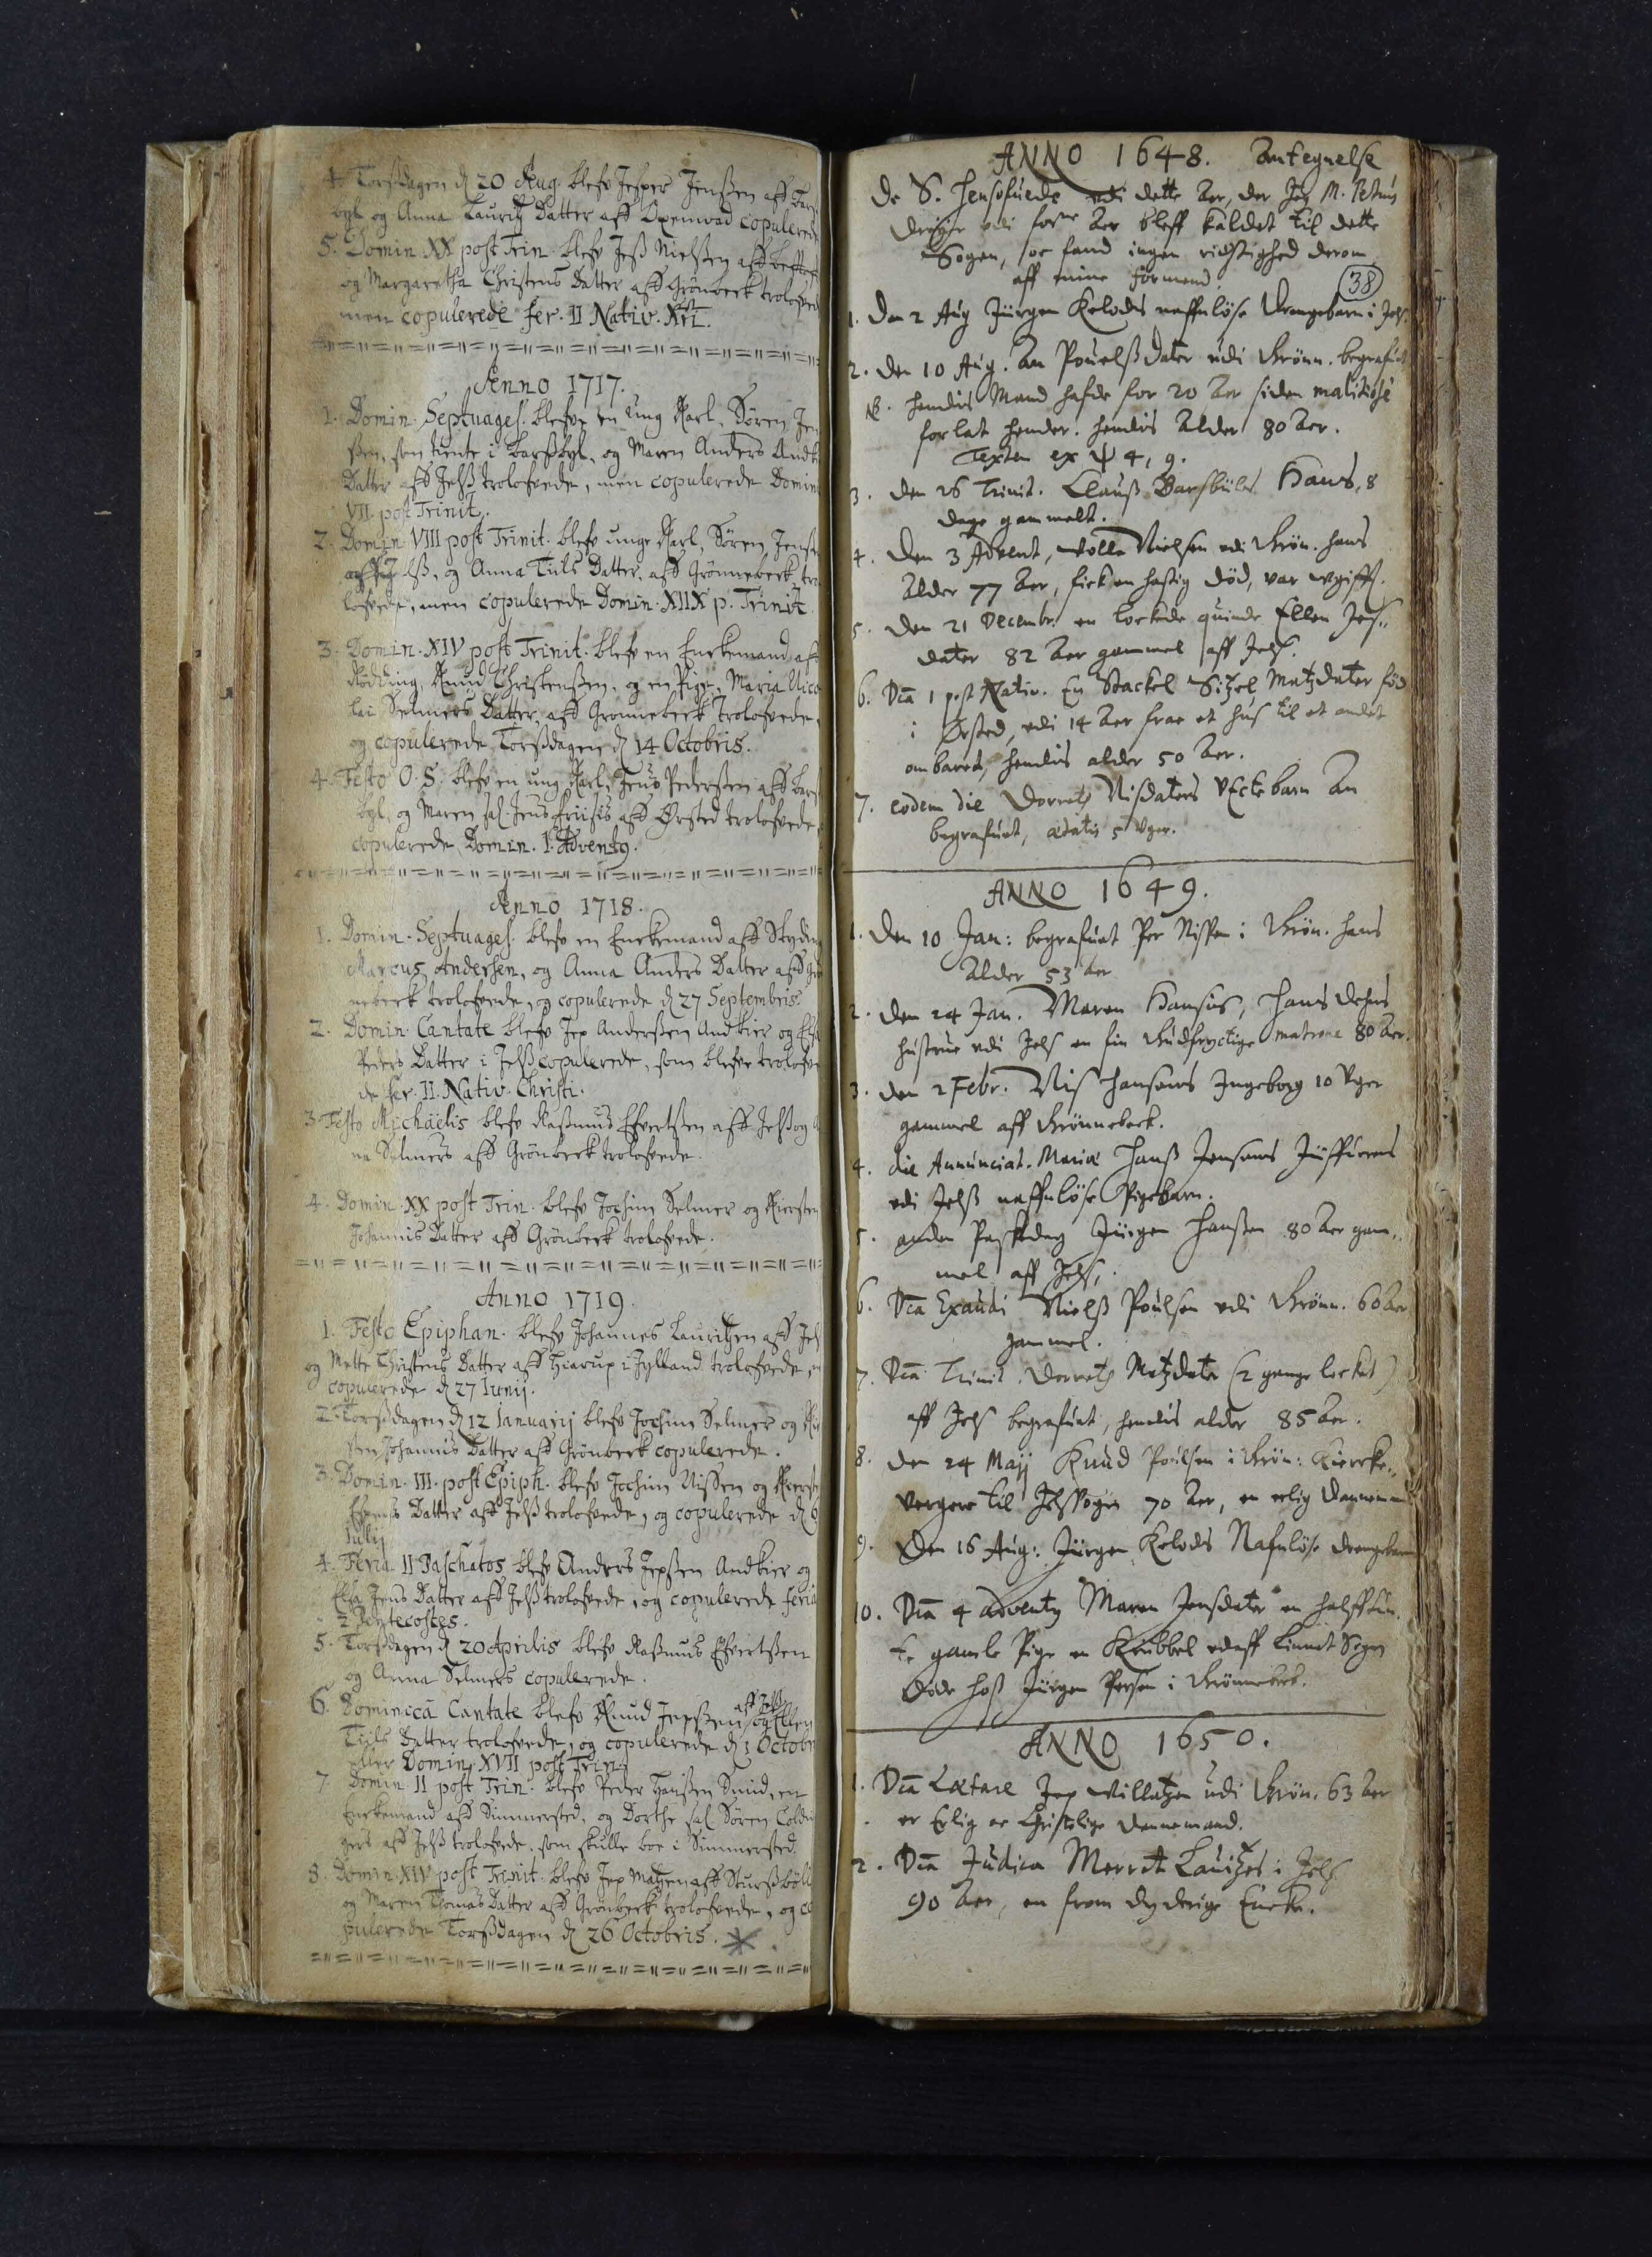4. Torssdagen d 20 Aug. blefv Jesper Jenssen aff Bars
byl og Anna Lauritz Datter aff Oxemvad copulerede
5. Domin. XX post Trin blefv Jess Nielssen aff Befftoft
og Margaretha Christens Datter aff Grønbeck trolofede
men copulerede fer. II Nativ. Xti.
Anno 1717.
1. Domin. Septuages. Blefve en ung Karl, Søren Jen
ssen, som tiente i Barssbyl, og Maren Anders Andki 
Datter aff Jelss trolofede, men copulerede Domin
VII post Trinit.
2. Domin. VIII post Trinit. blefv unge Karl, Søren Jenssen
aff Jelss, og Anna Tiils Datter, aff Grønnebeck tro
lofvede, men copulerede Domin. XIIX p. Trinit.
3. Domin. XIV post Trinit. blefv en Enckemand af 
Rødding, Knud Christenssen, og en Pige, Maria Nico¬
lai Selmers Datter, aff Grønnebeck trolofvede
og copulerede Torssdagen d 14 Octobris.
4. Festo O. S. blefv en ung Karl, Jens Pederssen aff Barss
byl, og Maren sal. Jens Friisis aff Ørsted trolofede,
copulerede Domin. I Adventq.
Anno 1718.
1. Domin. Septuages. blefv en Enckemand aff Styding
Marcus Andersen, og Anna Anders Datter aff Grøn
nebeck trolofvede, og copulerede d 27 Septembris.
2. Domin. Cantate blefv Jep Anderssen Andkier og Elsa
Peders Datter i Jelss copulerede, som blefve trolofve
de fer. II Nativ. Christi
3. Festo Michaelis blefv Rassmus Efvertssen aff Jelss og A
na Selmers aff Grønbeck trolofvede.
4. Domin. XX post Trin. blefv Jochim Selmers og Kiersten
Johannis Datter aff Grønbeck trolofvede.
Anno 1719.
1. Festo Epiphan. blefv Johannes Lauritzen aff Jels 
og Mette Christens Datter aff Hiarup i Jylland trolofvede
copulerede d 27 Iunij.
2. Torssdagen d 12 Ianuarij blefv Jochim Selmer og Kie
sten Johannis Datter aff Grønbeck copulerede.
3. Domin. III post Epiph. blefv Jochim Nissen og Kierste 
Datter aff Jelss trolofvede, og copulerede d 6
Iulij
4. Feria II Paschatos blefv Anders Jepssen Andkier og
Elsa Jens Datter aff Jelss trolofvede, og copulerede feria
2 Pentecostes.
5. Torssdagen d 20 Aprilis blefv Rassmus Efvertsen
og Anna Selmers copulerede.
aff Jelss
6. Dominica Cantate blefv Knud Jepssen og Ellen
Tiils Datter trolofvede, og copulerede d 1 Octobr 
eller Domin. XVII post Trinit.
7. Domin. II post Trin. blefv Peder Hanssen Smid, en
Enckemand aff Simmersted, og Dorthe sal Søren Coldi 
ger aff Jelss trolofvede, som skulle boe i Simmersted.
8. Domin. XIV post Trinit blefv Jep Matzen aff Sturssbøll
og Maren Thomas Datter aff Grønbeck trolofvede, og co
pulerede Torssdagen d 26 Octobris.
38
ANNO 1648. Antegnelse
De S. hensofuede udi dette Aar, der Jeg M Petrus
Dreyer vdi forne Aar bleff kaldet til dette
Sogen, oc fand ingen ##tighed derom
aff mine formend.
1. Den 2 Aug Jurgen Kelods naffnløse Drengebarn i Jels.
2. Den 10 Aug. An Pouelss Dater udi Grønn. Begrafuet.
NB. Hendis Mand hafde for 20 Aar siden malisiose
forlat hende. Hendis Alder 80 Aar.
Texten ex ψ 4, 9.
3. Den 26 Trinit. Clauss Barsbuler Hans, 8
Dage gammelt.
4. Den 3 Advent , Volle Nielsen vdi Grøn. hans
Alder 77 Aar, fick en hastig død, var vgift.
5. Den 21 Decembr. en lockede quinde Ellen Jes¬
dater 82 Aar gammel aff Jels.
6. Dca 1 post Nativ. En stackel Sitzel Matzater fød
i Ørsted, vdi 14 Aar frae et hus til et andet
om baret, hendis alder 50 Aar.
7. eodem die Dorreth Nisdaters vecte barn An
begrafuet, atatis 5 Vger.
ANNO 1649.
1. Den 10 Jan: begrafuet Per Nissen i Grøn. hans
Alder 53 Aar
2. Den 24 Jan. Maren Hansis, Hans Dehns
hustrue vdi Jels er fin Gudfryctige matrone 80 Aar.
3. Den 2 Febr. Nis Hansens Ingeborg 10 Vger
gammel aff Grønnebeck.
4. die Annunciat Maria Hans Jensens Jüffierens
vdi Jelss naffnløse Pigebarn.
5. anden Paskedag Jurgen Hanssen 80 Aar gam¬
mel aff Jels.
6. Dca Exaudi Nielss Poulsen vdi Grønn. 60 Aar
gammel.
7. Dca Trinit. Dorreth Matzdater (2 gange locket)
aff Jels begrafuet, hendis alder 85 Aar
8. Den 24 Maij Knud Poulsen i Grøn: Kiercke¬
verger til Jelssogn 70 Aar, en erlig Dannemand.
9. Den 16 Aug: Jurgen Kelods Nafnløse drengebarn
10. Dca 4 adventq Maren Jensdater en
gamle Pige oc Krübbel vdaff Linnet Sogn
døde hos Jurgen Persen i Grønnebeck.
ANNO 1650.
1. Dca Latare Jep Villatzen udi Grøn, 63 Aar
en Erlig oc Christlige Dannemand.
2. Dca Judica Merret Lauitzes i Jels
90 Aar, en from dyderige Encke.
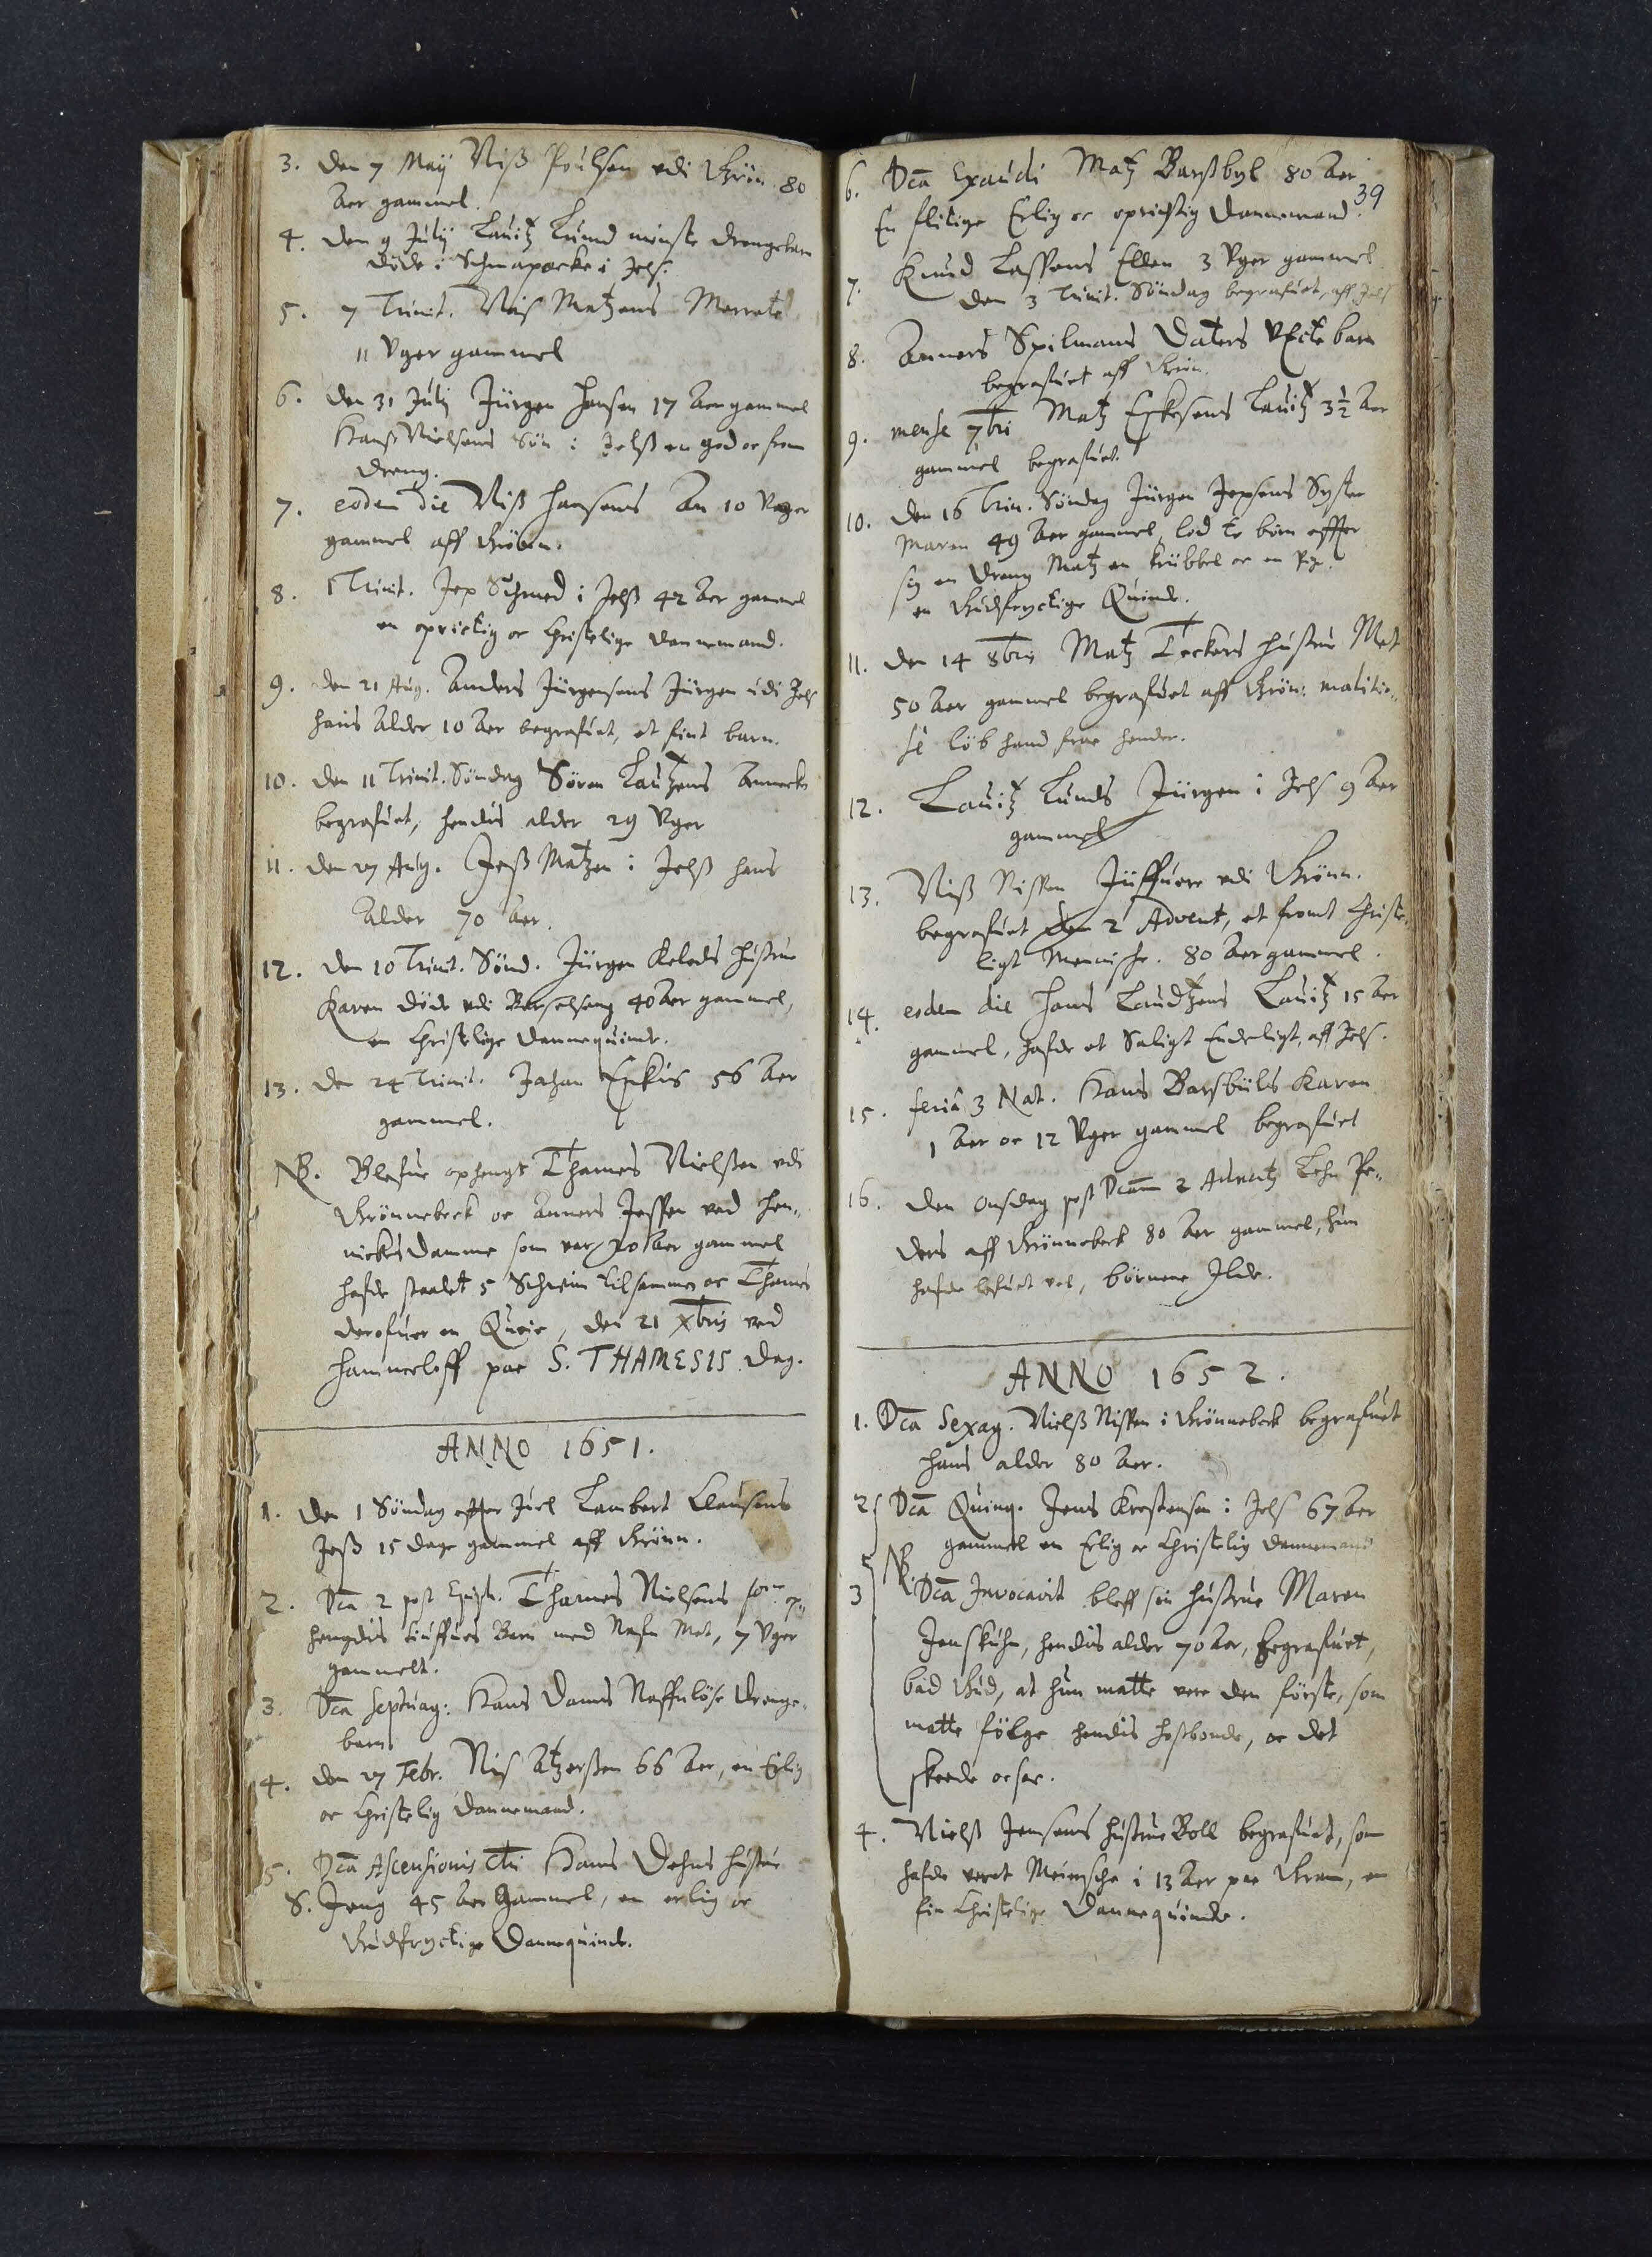3. Den 7 Maij Niss Poulsen vdi Grøn 80
Aar gammel.
4. Den 9 Julij Lauitz Lund minste Drengebarn
døde Schmapacke## i Jels
5. 7 Trinit. Nis Matzens Merrete
11 Vger gammel
6. Den 31 Julij Jurgen Hansen 17 Aar gammel
Hans Nielsens søn i Jelss en god oc from
dreng.
7. eodem die Niss Hansens An 10 Vger
gammel aff Grønn
8. 1 Trinit Jep Schmed i Jelss 42 Aar gammel
en oprictig oc Christelige Dannemand.
9. Den 21 Aug. Anders Jurgensens Jurgen udi Jels
hans Alder 10 Aar begrafuet, et fint barn.
10. Den 11 Trinit. Søndag Søren Lautzens Anneke
begrafuet, hendis alder 29 Vger.
11. Den 27 Aug. Jess Matzen i Jelss hans
Alder 70 Aar
12. Den 10 Trinit. Sønd. Jurgen Kelods Hustrue
Karen døde vdi Barselseng 40 Aar gammel,
en Christelige Dannequinde.
13. Den 24 Trinit. Jahan Eskis 56 Aar
gammel.
NB. Blefue ophengt Thames Nielssen vdi
Grønnebeck oc Anners Jessen ved Hen¬
nickesdamme som var 20 Aar gammel
hafde staalet 5 Schvin tilsammen oc Thames 
derofuer en Queie, den 21 xtris ved 
Hammeleff paa S. THAMESIS. Dag.
ANNO 1651
1. Den 1 Søndag efter Juel Lambert Clausens
Jess 15 Dage gammel aff Grønn.
2. Dca 2 post Epiph. Thames Nielsens som
op-
hengdis tiuffuers Barn med Nafn Met, 7 Vger
gammelt.
3. Dca Septuag: Hans Dams Naffnløse Drenge¬
barn.
4. Den 27 Febr. Nis Atzerssen 66 Aar, en Erlig
oc Christelig Dannemand.
5. Dca Ascensionis Hans Dehns hustrue
S. Jeng 45 Aar gammel, en erlig oc
Gudfryctige Dannequinde.
39
6. Dca Exaudi Matz Barssbyl 80 Aar
en flitige Erlig oc oprichtig Dannemand.
7. Knud Lassens Ellen 3 Vger gammel
den 3 Trinit. Søndag begrafuet, aff Jels
8. Anners Spilmans Daters VEcte barn
begrafuet aff
9. mense 7tri Matz Eskesens Lauitz 3½ Aar
gammel begrafuet.
10. Den 16 Trin. Søndag Jurgen Jepsens Syster
Maren 49 Aar gammel, lod to børn efter
sig en dreng Matz en krübbel oc en pige.
en Gudfryctige Quinde
11. Den 14 8tris Matz Teckers hustrue Met
50 Aar gammel begrafuet aff Grøn: malitio¬
si løb hand frae hende.
12. Lauitz Lunds Jurgen i Jels 9 Aar
gammel
13. Niss Nissen Juffuer vdi Grønn.
Begrafuet den 2 Advent, et fromt Christe¬
ligt menniske. 80 Aar gammel.
14. eodem die Hans Laudtzens Lauitz 15 Aar
gammel, hafde et Saligt Endeligt, aff Jels.
15. feria 3 Nat. Hans Barsbuls Karen
1 Aar oc 12 Vger gammel begrafuet.
16. Den Onsdag post Dcum 2 Adventq Lehn Pe¬
ders aff Grønnebeck 80 Aar gammel, hun
hafde lefuet vel, børnene Ilde
ANNO 1652.
1. Dca Sexag. Nielss Nissen i Grønnebeck begrafuet
hans alder 80 Aar
2. Dca Quing. Jens Krestensen i Jels 67 Aar
gammel en Erlig oc Christelig Dannemand
3. Dca Invocavit bleff sin hustrue Maren
Jens kuhn, hendis alder 70 Aar, begrafuet
Bad Gud, at hun matte være den første, som
matte følge hendis husbonde, oc det
skeede ocsae.
4. Niels Jensens hustrue Boll bgrafuet, som
hafde været Meirsche i 13 Aar paa Gram, en 
fin Christelige Dannequinde.